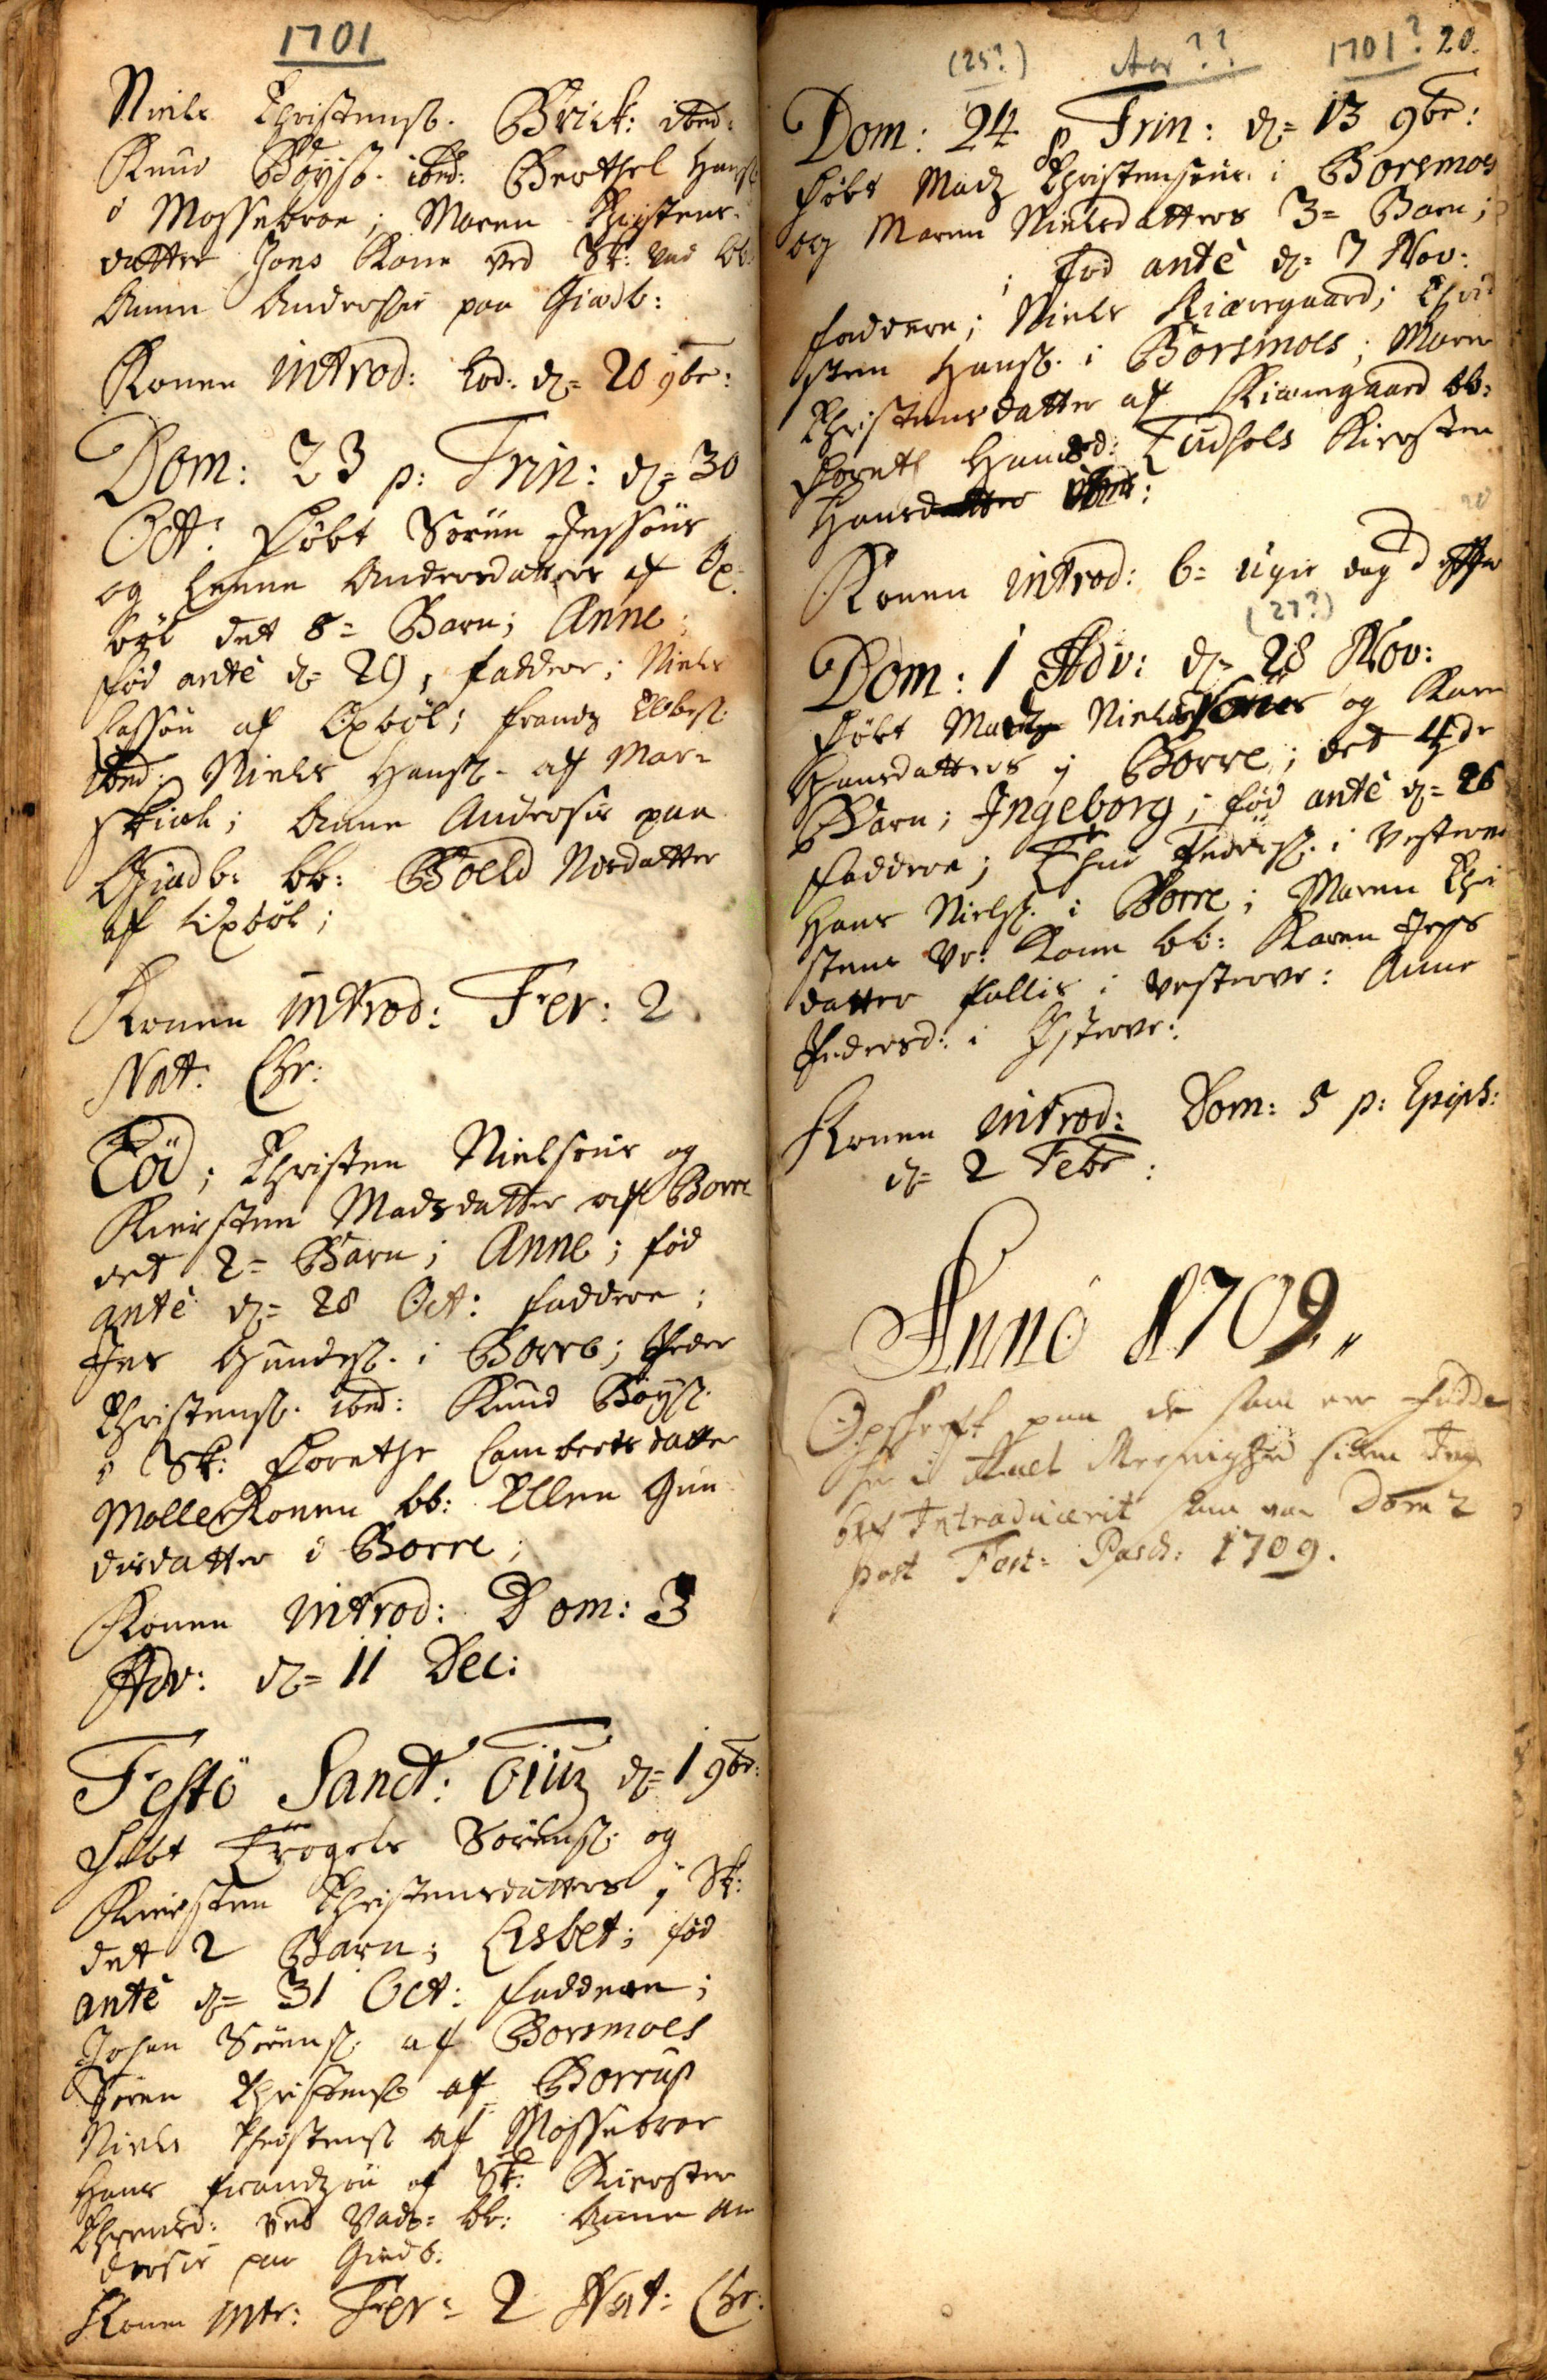1701
Niels Christens Brick: ibm:
Knud Boys ibm:, Berthel Hans.
i Mossebroe; Maren Christens¬
datter Jons Kone ved Sk: Vad bb:
Anne Andersis paa Giedb:
Konen introd: Eod. d= 20 9br:
Dom: 23 : p: Trin: d= 30
Oct: Døbt Søren Jessøns
og Leene Andersdatters af Ox¬
bøl det 5= Barn; Anne;
fød ante d=  .29;  Faddere; Niels
Lassøn af Oxbøl; Frandz Ebbes
ibm: Niels Hans af Mar¬
skiæl; Anne Andersis paa
Giædb: bb: Boeld Nisdatter
af Oxbøl;
Konen introd: Fer: 2.
Nat: Chr:
Eod; Christen Nielsøns og
Kiersten Madzdatter af Borre
det 2= Barn; Anne; fød
ante d= 28 Oct: Faddere;
Jes Gundes i Borre; Peder
Christens ibm: Knud Boys
i Sk: Dorethe Lambertsdatter
Møller Konen bb: Ellen Gun¬
disdatter i Borre;
Konen introd: Dom: 3
Adv: d.11 Dec:
Festo Sanct: Tirs d=1 9br:
Døbt Trogels Sørens og
Kiersten Christensdatters j Sk:
det 2 Barn; Lisbet; fød
ante d= 31 Oct: Faddere;
Johan Sørensen af Børsmoes,
Søren Christens af Borrup
Niels Christens af Mossebroe
Hans Frandzeøn af Sk: Kiersten
Chrensd: ved Vad: bb: Anne An¬
dersis paa Giedb:
Konen intr: Fer: .2 Nat: Chr.
20
Dom: 24 p Trin: d= 13 9br:
Døbt Madz Christensøns i Børsmos
og Maren Nielsdatters 3= .Barn;
; fød ante d= 7 Nov:
Faddere; Niels Kiæregaard; Chri¬
sten Hans i Børsmoes; Maren
Christensdatter af Kiæregaard bb:
Doreth Hansd: Tudhols Kiersten
Hansdatter ibm:
Konen introd. 6. Ugis dag derefter
Dom:1 Adv: d= 28 Nov:
Døbt Madz Nielsøns og Karen
Hansdatters j Borre det 4de
Barn; Ingeborg; fød ante d= 26.
Faddere; Thue Peders i Vester##
Hans Niels i Borre; Maren Chri¬
sten Vr. Kone bb: Karen Jeps
datter Pallis i Vestervr: Anne
Pedersd: i Østervr:
Konen introd: Dom.5 : .Epiphan:
d= 2 Febr:
Anno 1709.
Opskrift paa den ## ## ##
her i Aael Meenighed si## ##
blef Introducerit ## var Dom 2
post ## Pasch: 1709
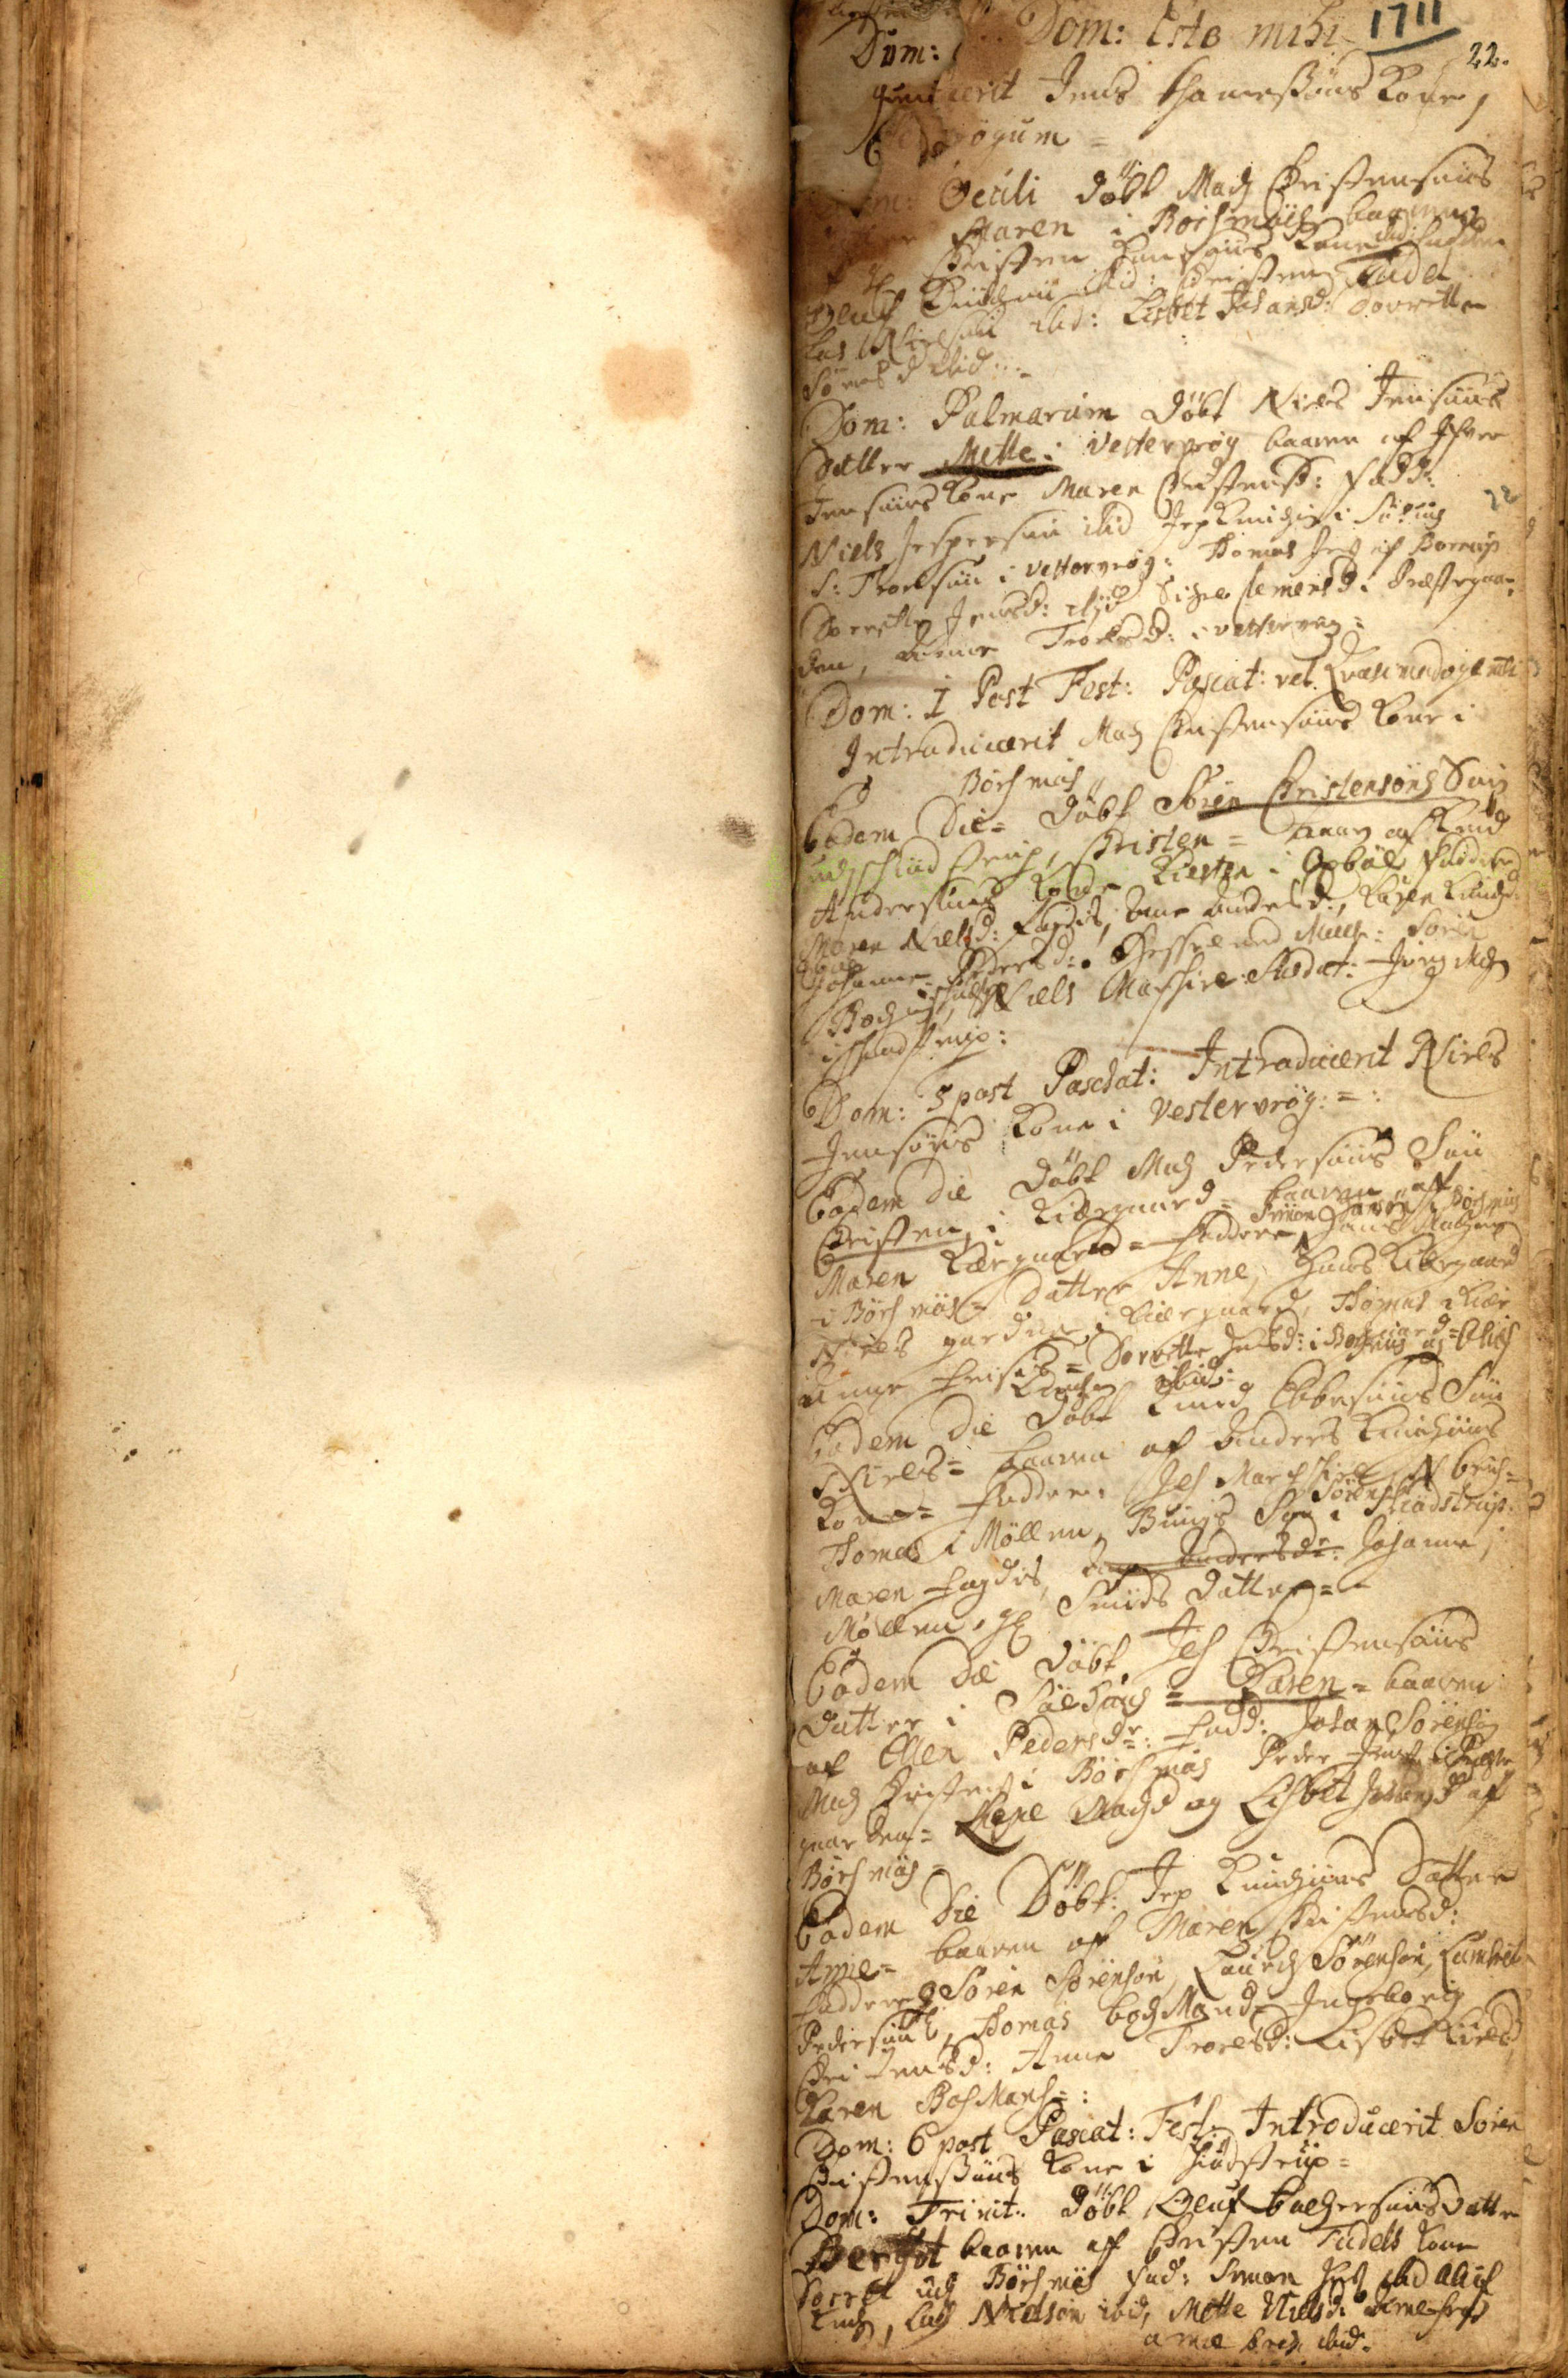22
22
1711
Dom. Esto Mihi
##erit Jens Hansøns Kone,
##øgum=
Dom: Oculi døbt Mads Christensens
## Karen i Børsmoes ##
Christen Hansøns Kone ##:  Faddere
Oluf ## ibid: Christen kaldet
Las Nielsøn ibid Lisbet Johans: Dorrette
Sørensd ibid
Dom: Palmarum døbt Niels Jensøns
Datter Mette i Vestervrøg. baaren af Ifver
Jensøns Kone Maren Christensd: Fadd:
Niels Jespersøn ibid Jep Knudsøn i Søhuus
S: Troelsøn i Vestervrøg: Thomas Jess af Borrup
Dorethe Jensd: ibid Sidsel Clemensd. i Præstegaar¬
den, Anne Troelsd: i Vestervrøg:
Dom: 1 Post Fest: Pascat. ## Qvasimodogeniti
Introducerit Madz Christensøns Kone i
Børsmos
Eodem Die= døbt Søren Christensøns Søn
udi Schiødstrup, Christen=- baaren af Knud
Andersøns Kone Kiersten i Oxbøl Faddere
Maren Nielsd: Fogdet, Anne Andersd:, Karen Lauridsd
Johanne Pedersd: Hesselmed Møll:, Søren
Bods Schiødstr Niels Marskiel: ##, Jørg ##
i Schiødstrup:
Dom: 5 post Paschat: Introducerit Niels
Jensøns Kone i Vestervrøg: = :
Eodem Die døbt Madz Pedersøns Søn
Christen i Kiærgaard= baaren af
Simon Hansøn i Børsmos
Maren Kærgaard= Faddere: Hans ##.
i Børsmos=  Datter Anne, Hans Kiærgaard
Niels garder i Kiærgaard, Thomas  Kiær##
Anne Frises= Dorette Jensd: i Børsmos og = Oluf
Knudsen ibid:
Eodem Die døbt Knud Ebbesøns Søn
Niels= baaren af Anders Knudsøns
Kone= Faddere: Jes Marschiel, N Brich=
Søren
Thomas i Møllen, Bundis Søn i Schiødstrup
Maren Fogdis, ## Johanne j
Møllen, gl Smids Datter=
Eodem Die døbt Jes Christensøns 
datter i Søehuus= Karen= baaren
af Ellen Pedersdr: Fadd: Johan Sørensøn
Madz Christenbs i Børsmos, Peder Jens i ##
gaarden= Lene Madzd og Lisbet Johansd af
Børsmos=
Eodem Die Døbt Jep Knudzøns Datter
Anne= baaren af Maren Christensd:
Faddere ## Søren Sørensøn, Lauridz Sørensøn, Lambert
Pedersøn ## Thomas BodzMand= Ingeborig
Christensd: Anne Troelsd: Lisbet Kieldsd##
Karen Bosmans##= :
Dom. 6 post Pascat. Fest. Introducerit Søren
Christensøns Kone i Schiødstrup.
Dom: Trinit: døbt Oluf Bachensens Datter
Derett, baaren af Christen Tudels Kone
Dorret af Børsmos. Fad: Simon Jes ibid ##
##, Las Nielsen ibid, Mette Nielsd. Anne Fogd##
Anne## Brich## ibid.